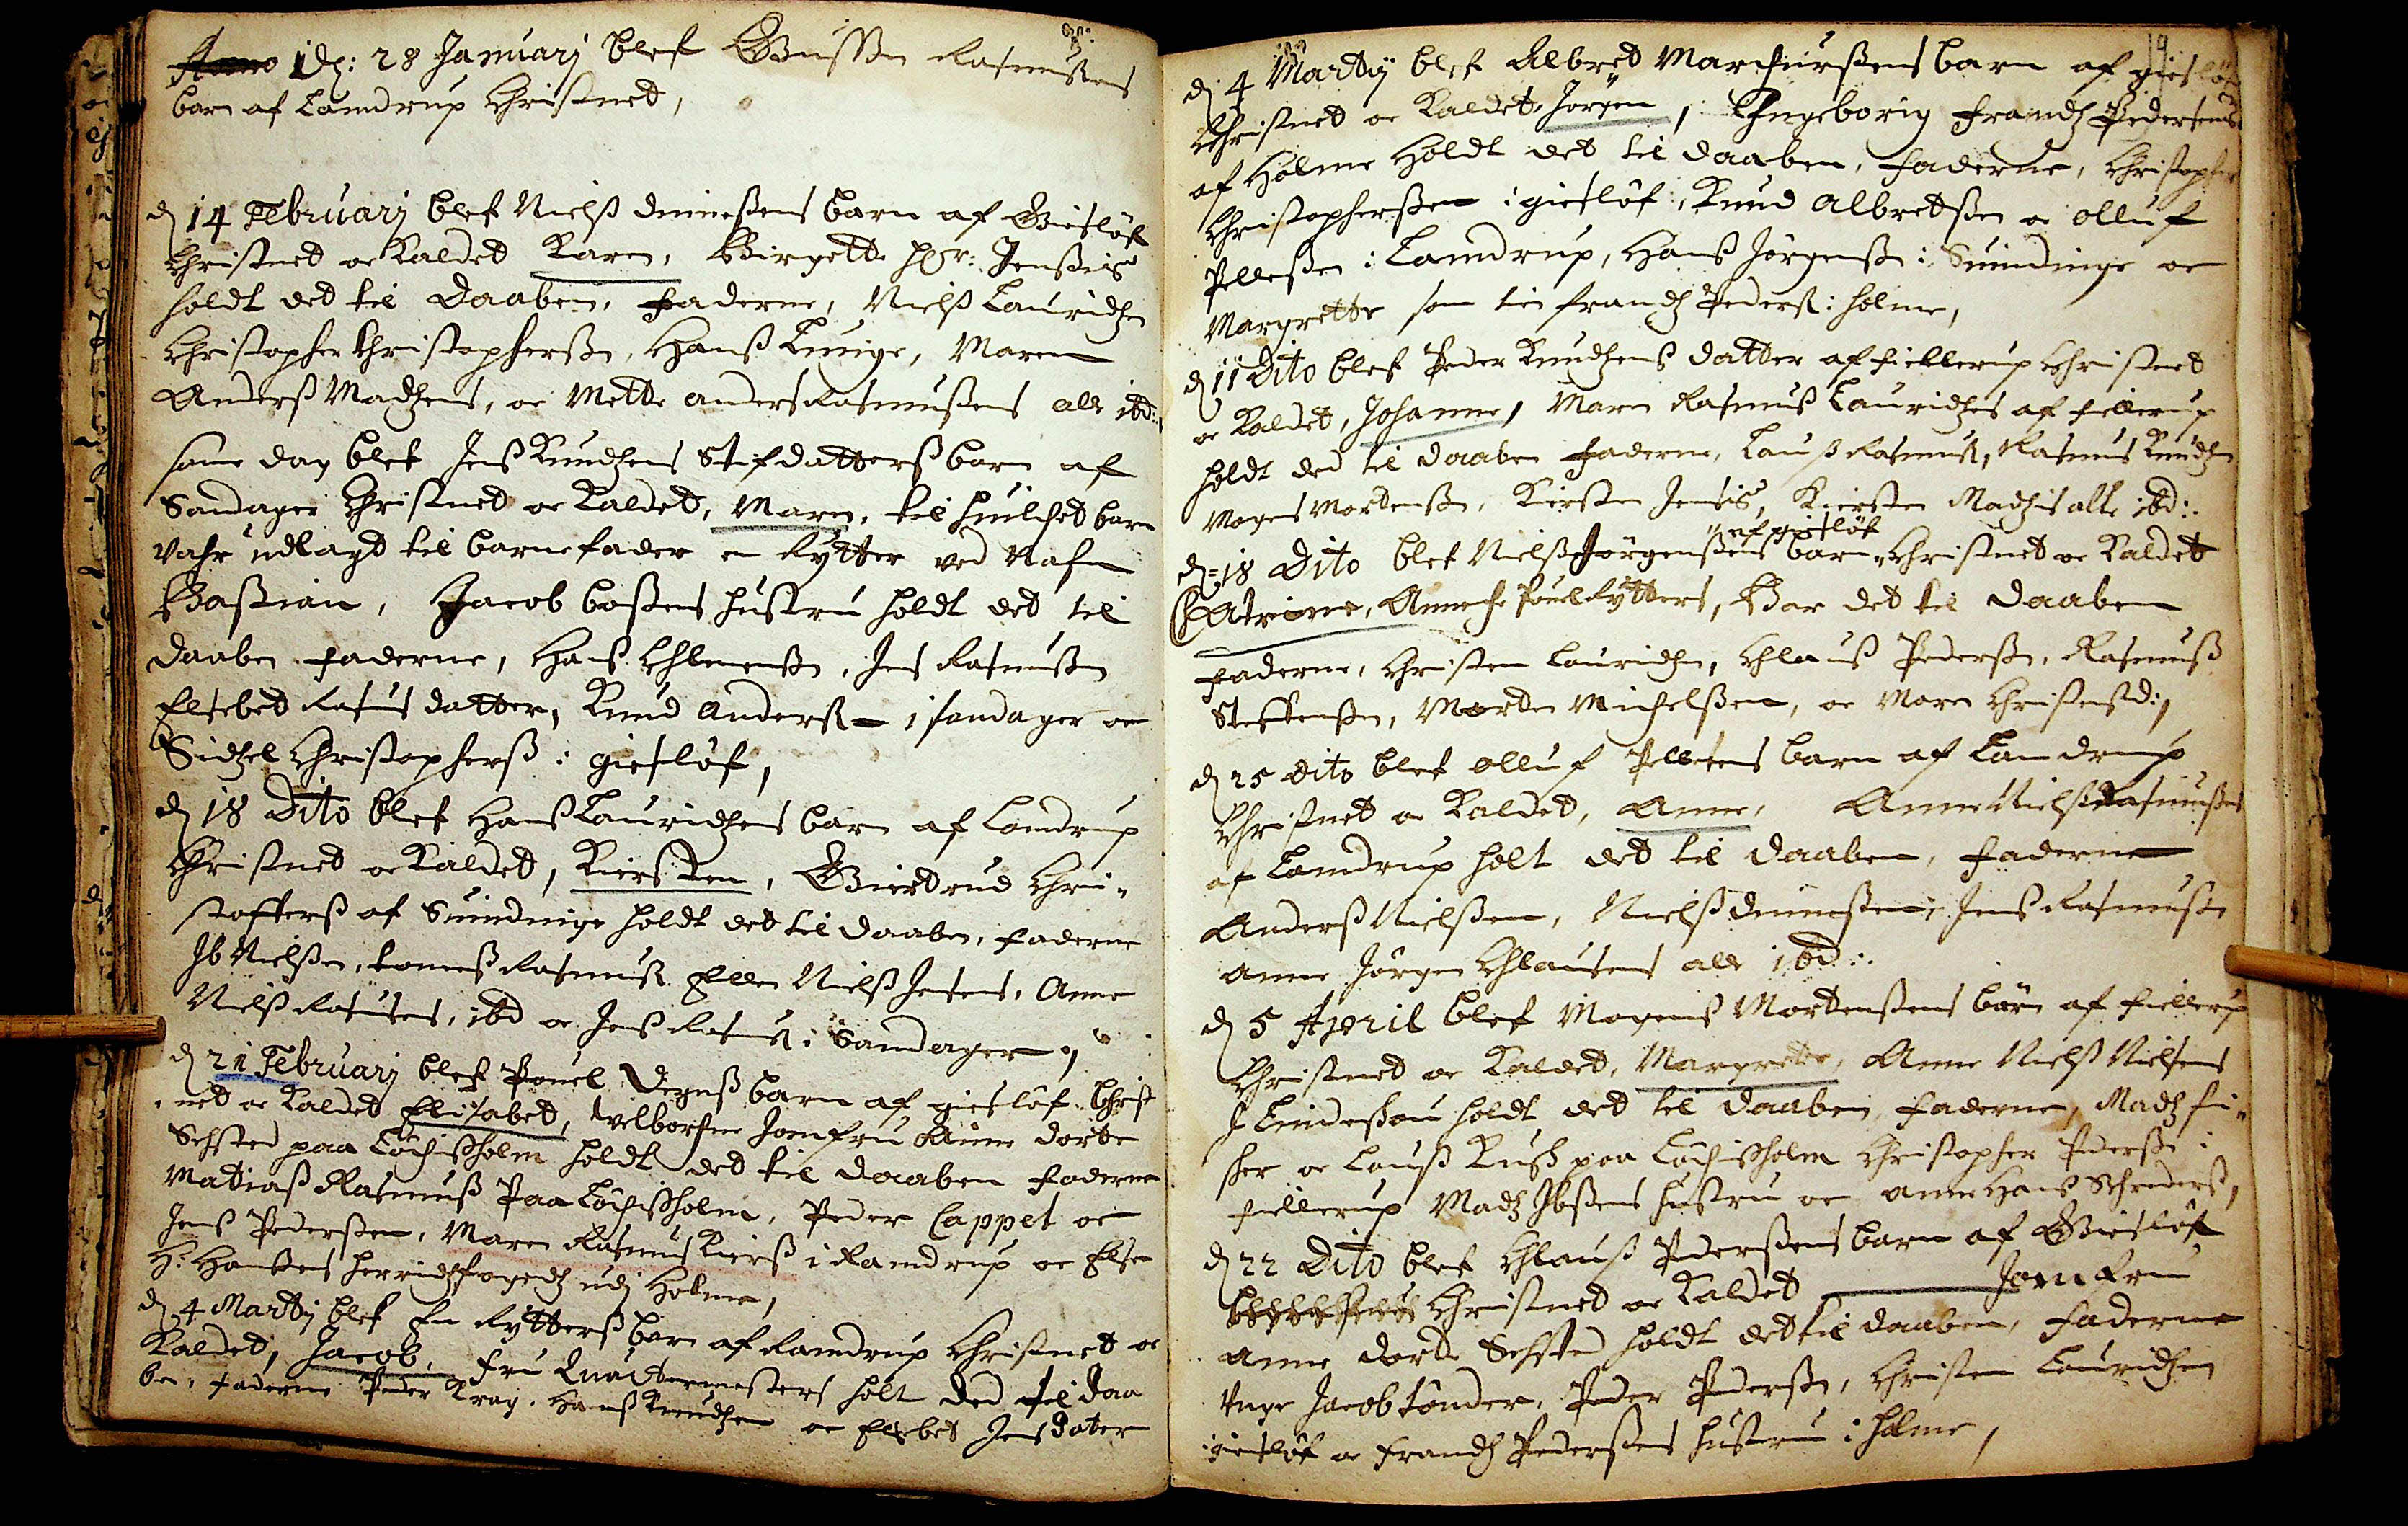Anno d: 28 Januarij blef Gus## Rasmussens
barn af Lamdrup Christnet
d 14 Februarij blef Niels Dinnessens barn af Giesløf
Christned oc kaldet Karen, Birgette Hlr: Jensis
holdt det til Daaben. Faderne, Nielss Lauridtzen
Christopher Christopherss, Hanss Lunige, Maren
Anders Madtzens, oc Mette Anders Rasmussens alle ibd:.
same dag blef Jenss Knundtzens Stifdatters barn af
Sandager Christned oc kaldet, Maren, til huilchet barn
vahr udlagt til barnefader en Rytter ved Nafn
Bastian, Jarob bossens hustru holdt det til
daaben. Faderne, Hanss Chlemenssen, Jens Rasmussen
Elsebet Rasmusdatter, Knud Anders - i sandager oc
Sidtzsel Christophers i Giesløf
d 18 Dito blef Hans Lauridtzes barn af Lamdrup
christned oc kaldet Kiersten, Giertrud Chri¬
stofferss af Suindinge holdt det til daaben, Faderne
Ib Nielssen , Tomess Rasmuss Ellen Nielss Jensens, Anne
Nielss Rasmussens, ibd oc Jenss Rasmus i Sandager,
d 21 Februarij blef Pouel Degnss barn af giesløf Christ
= net oc kaldet Elisabet, velborhne Jomfru Ane dorte
Sehsted paa Løchissholm holdt det til daaben Faderne
Mattiass Rasmuss Paa Løchissholm, Peder Cappet oc
Jens Pederssen, Maren Rasmus Kierss i Ramdrup oc Else
H: Hanssens herridtzfogedtz udi Holme
d 4 Martij blef Een Rytterss barn af Ramndrup Christenet oc
kaldet, Jarob, fru Quartermesters holt ved til daa
ben Faderne Peder Krag Hans Knudtzen oc Elsebetl Jensdater
14.
d: 4 Martij blef Albret Marchurssens barn af giesløf
Christnet oc kaldet Jørgen, Engeborig, Frandtz Pedersens
af Holme holdt det til Daaben. Faderne, Christopher
Christopherssen i giesløf:, Knud Albredtssen oc Olluf
Pellessen i Lamdrup, Hanss Jørgenssen i Suindige oc
Margrette som tien Frandtz Peders i holme,
d 11 Dito blef Peder Knudtzens datter af Fiellerup Christnet
oc kaldet, Johanne, Maren Rasmuss Lauridtzens af Fiellerup
holdt ded til daaben Faderne, Lauss Rasmus, Rasmus Knudtzen
Mogens Moetenssen, Kiersten Jensis, Kiersten Madtzis alle ibd:.
af giesløf
d. 18 Dito blef Niels Jørgensens barn Christnet oc kaldet
Chatrine, Anneche Pouel Rytters, Bar det til Daaben
Faderne, Christen Lauridtzen, Chlauss Pederssen, Rasmuss
Steffenssen, Morten Michelssen, oc Maren Christensd:,
d 25 Dito blef Olluf Pellesens barn af Lamdrup
Christnet oc kaldet, Anne, Anne Nielss Rasmussens
af Lamdrup holt det til Daaben, Faderne
Anderss Nielssen, Nielss Dinnesen, Jenss Rasmusen
Anne Jørgen Chlausens alle ibd:.
d 5 April blef Mogens Mortenssens børn af Fiellerup
Christnet oc kaldet, Margrette, Anne Nielss Nielsens
I Lindeskou holdt det til daaben, Faderne, Madz fi¬
sher oc Lauss Kush paa Løchissholm Christopher Pederssen i
fiellerup Madtz Ibssens hustru oc Anne Hans Schreders,
d 22 Dito blef Chlaus Pederssens barn af Giesløf
Bsbyffvrs Christned oc kaldet _ Jomfru
Anne Dorte Sehsted holdt det til daaben, Faderne
vnge Jacob tønder,. Peder Pederssen, Christen Lauridtzen
i giesløf oc Frandtz Pederssens hustru i Holme,
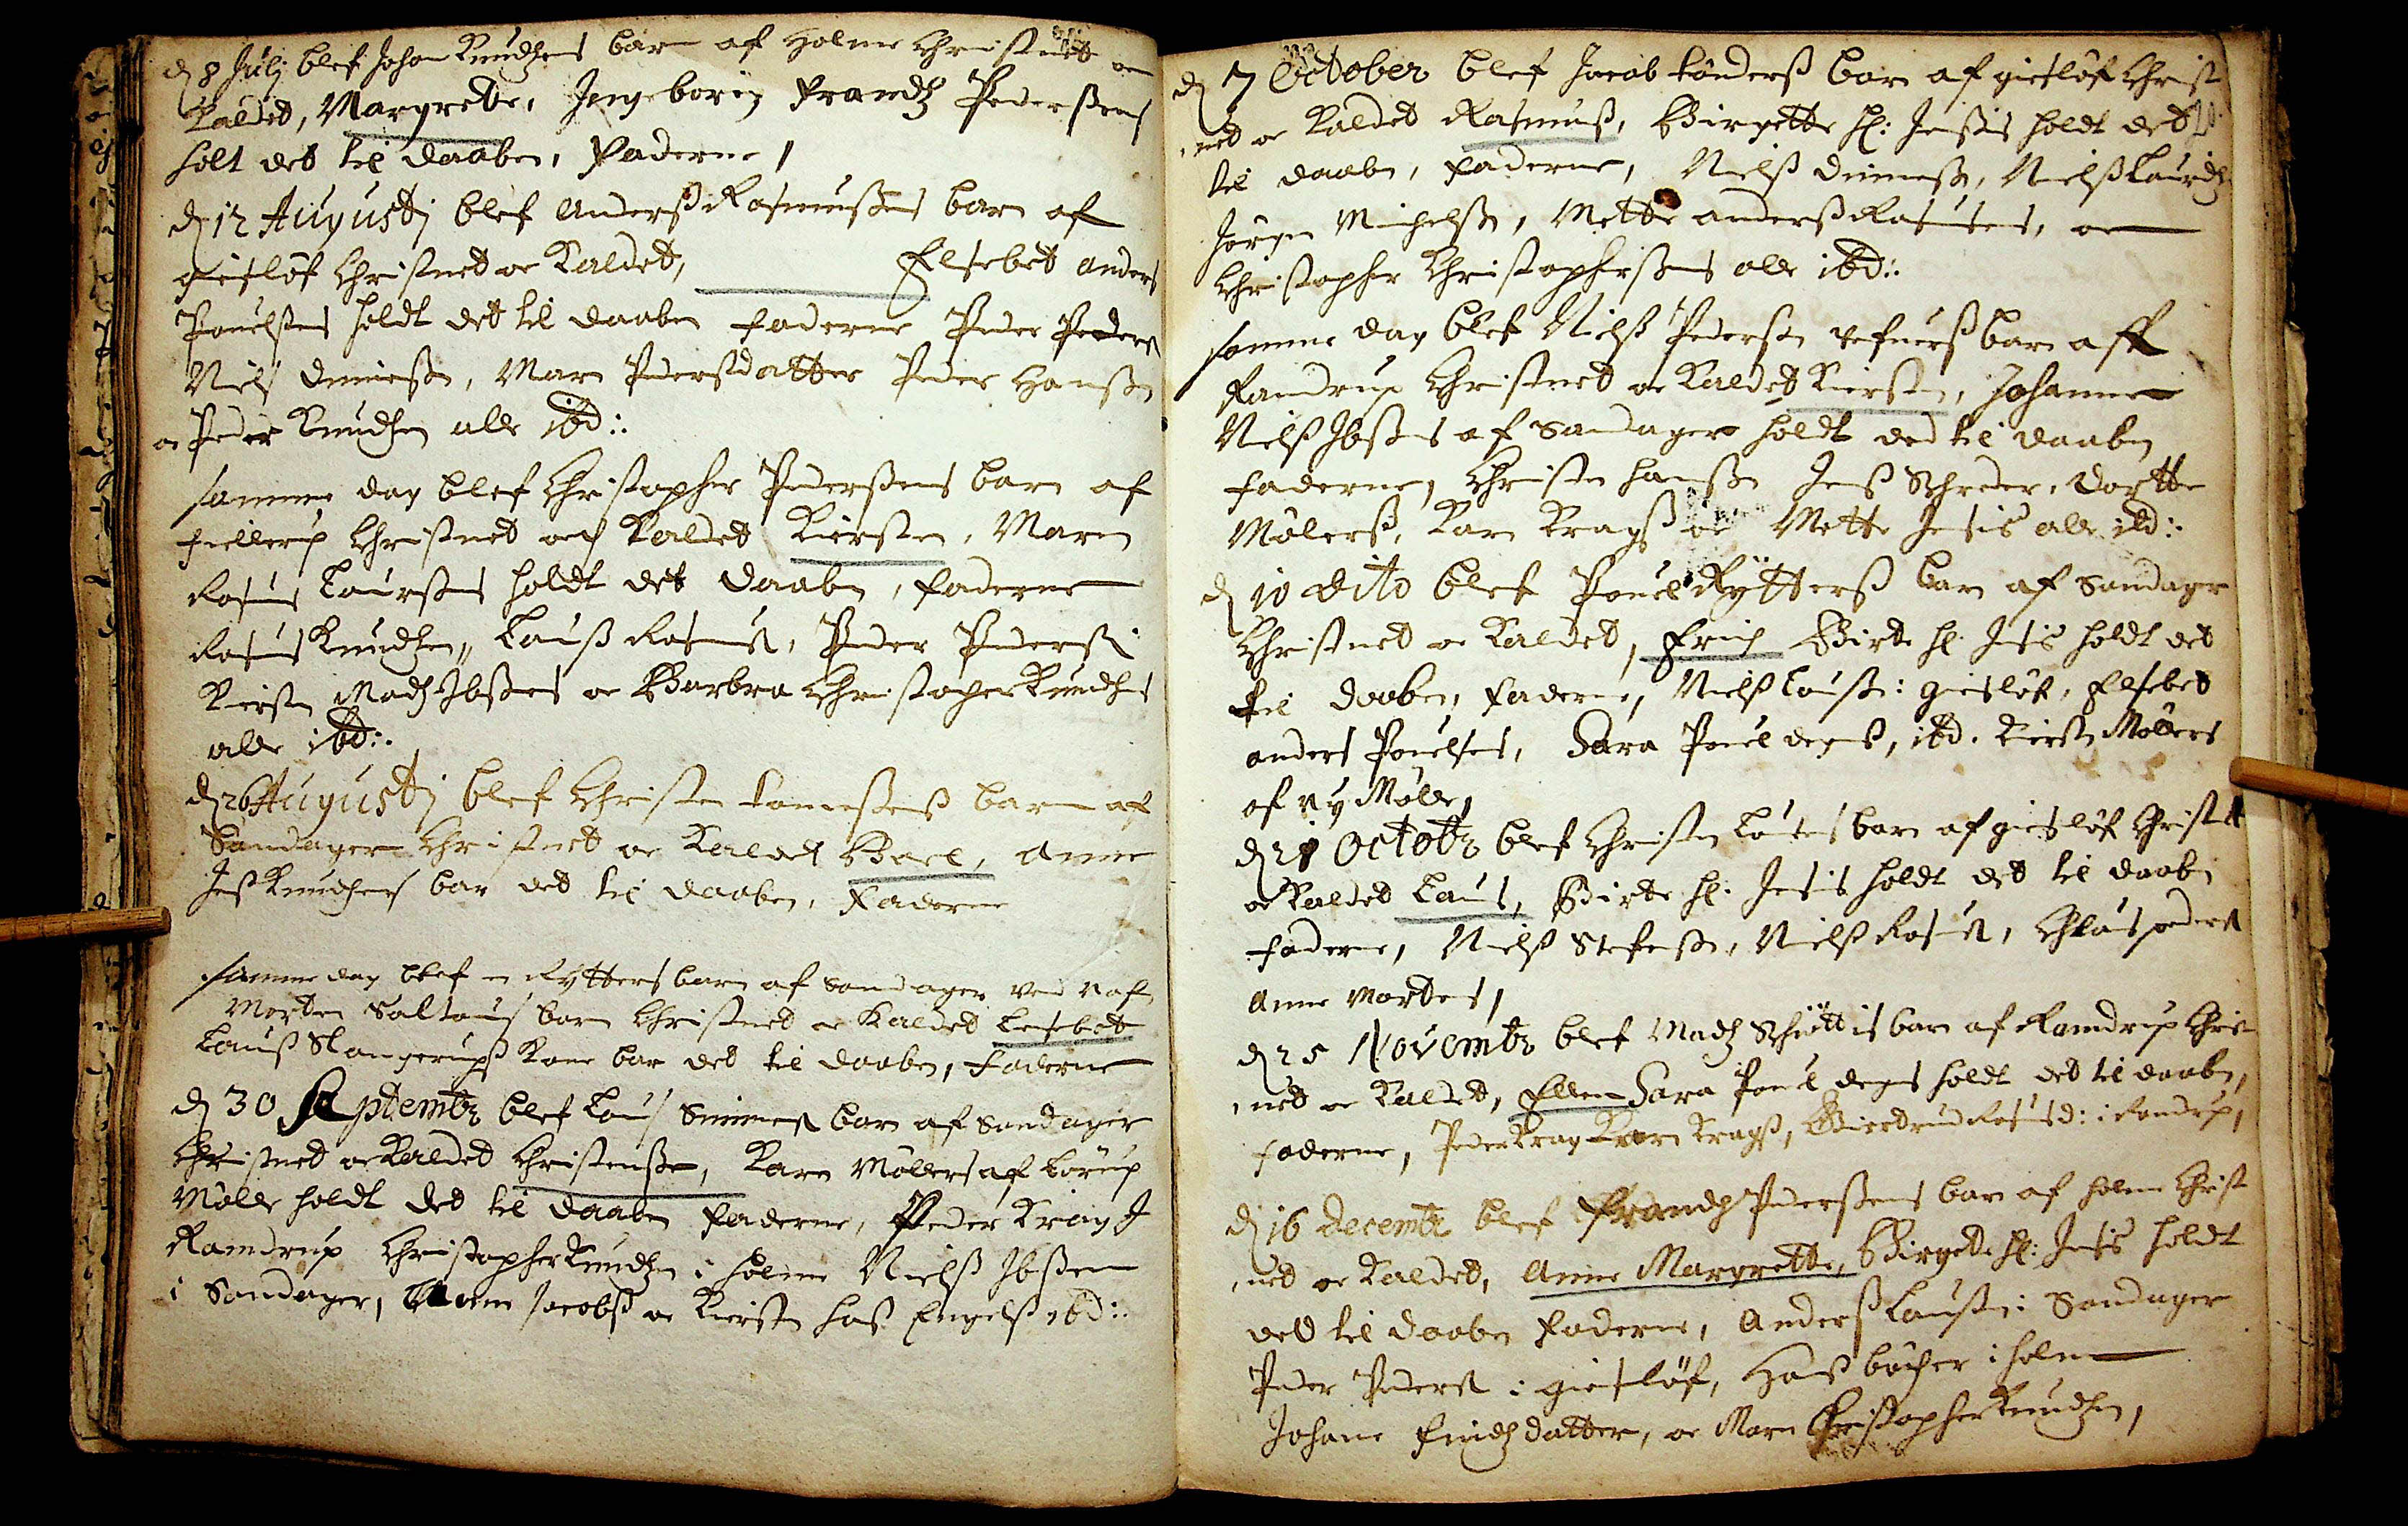d 8 Julij blef Johan Knudtzens barn af Holme Christner oc
kaldet, Margrette, Jengeborig Frandtz Pedersens
holt det til Daaben, Faderne
d 12 Augustij blef Anderss Rasmussens barn af
giesløf Christnet oc kaldet
Elsebek Anders
Pouelssens holdt det til daaben Faderne Peder Peders
Niels Dinnessen, Maren Pederssdatter Peder Hanssen
oc Peder Knudtzen alle ibd:.
samme dag blef Christopher Pederssens barn af
fiellerp Christned och kaldet Kiersten, Maren
Rasmus, Laurssens holdt det daaben, Faderne
Rasmus Knudtzen, Lauss Rasmus, Peder Peders
Kiersten Madtz Ibsens oc Barbra Christoper Knudtzens
alle ibd:.
d 26 Atugustij blef Christen Tomessenss barn af
Sandager Christenet oc kaldet Boel, Anne
Jenss Knudsens bar det til daaben, Faderne
Samme dag blef en Rytters barn af Sandager ved Naf
Morten Saltous barn Christnet oc kaldet Elsebet
Lauss Slangerups Kone bar det til daaben, Faderne
d 30 Septembr. blef Laus Sim##es barn af Sandager
Cristnet oc kaldet Christenssen, Karen Møllers af Lørup
Mølle holdt det til daaben Faderne, Peder Brag I
Ramdrup Christopher Knudtzen i Holme Niels Ibssen
i Sandager, Anne Jacobss oc Kiersten hos Engelss ibd:.
20.
d 7 October blef Jacob Tønderss barn af giesloff Christ
= net oc kaldet Rasmuss, Birgette Hl: Jenssis holdt det
til daaben, Faderne, Niels Dinness, Niels Laurdtz
Jørgen Michelss, Mette Anderss Rasmussens oc
Christopher Christopherssens alle ibd:.
Samme dag blef Nielss Pedersen Vefuerss barn aff
Randrup christnet oc kaldet Kiersten, Johanne
Niels Ibssens af Sandager holdt ded til daaben
Faderne, Christen Hanss Jens Schreder, Dortte
Mølerss, Karn Kragss Mette Jensis alle ibd:.
d 10 Dito blef Pouel Rytterss barn af Sandager
Christned oc kaldet Erich Birtte hl. Jensis holdt det
til daaben, Faderne Nielss Lauss: i Giesløf, Eleebet
anders Pouelsens, Sara Pouel degnss, ibd. Kierst Møllers
af ny Mølle,
d 21 Octobr. blef Christen Lausens barn af giesløf Christnet
oc kaldet Laus, Birte Hl. Jensis holdt det til daabn
Faderne, Niels Stefenss Niels Rasmus, Chlaus peders
Anne Mortens,
d 25 Novembr blef Madtz Schiøttis barn af Ramdrup Christ
= net oc kaldet, Ellen Sara Pouel degns holdt det til daabn,
Faderne, PederKrag Karn Kragss, Gierdrud Rasmusd: i Ramdrup
d 16 Decembr blef Frandtz Pederssens bar af holme Christ
= net oc kaldet, Anne Margrette, Birgete hl: Jensis holdt
det til daaben Faderne, Anderss Laussen i Sandager
Peder Peders i giesløf, Hanss bøcher i holme
Johane Findtzdatter, oc Marn Christopher Knudtzen,